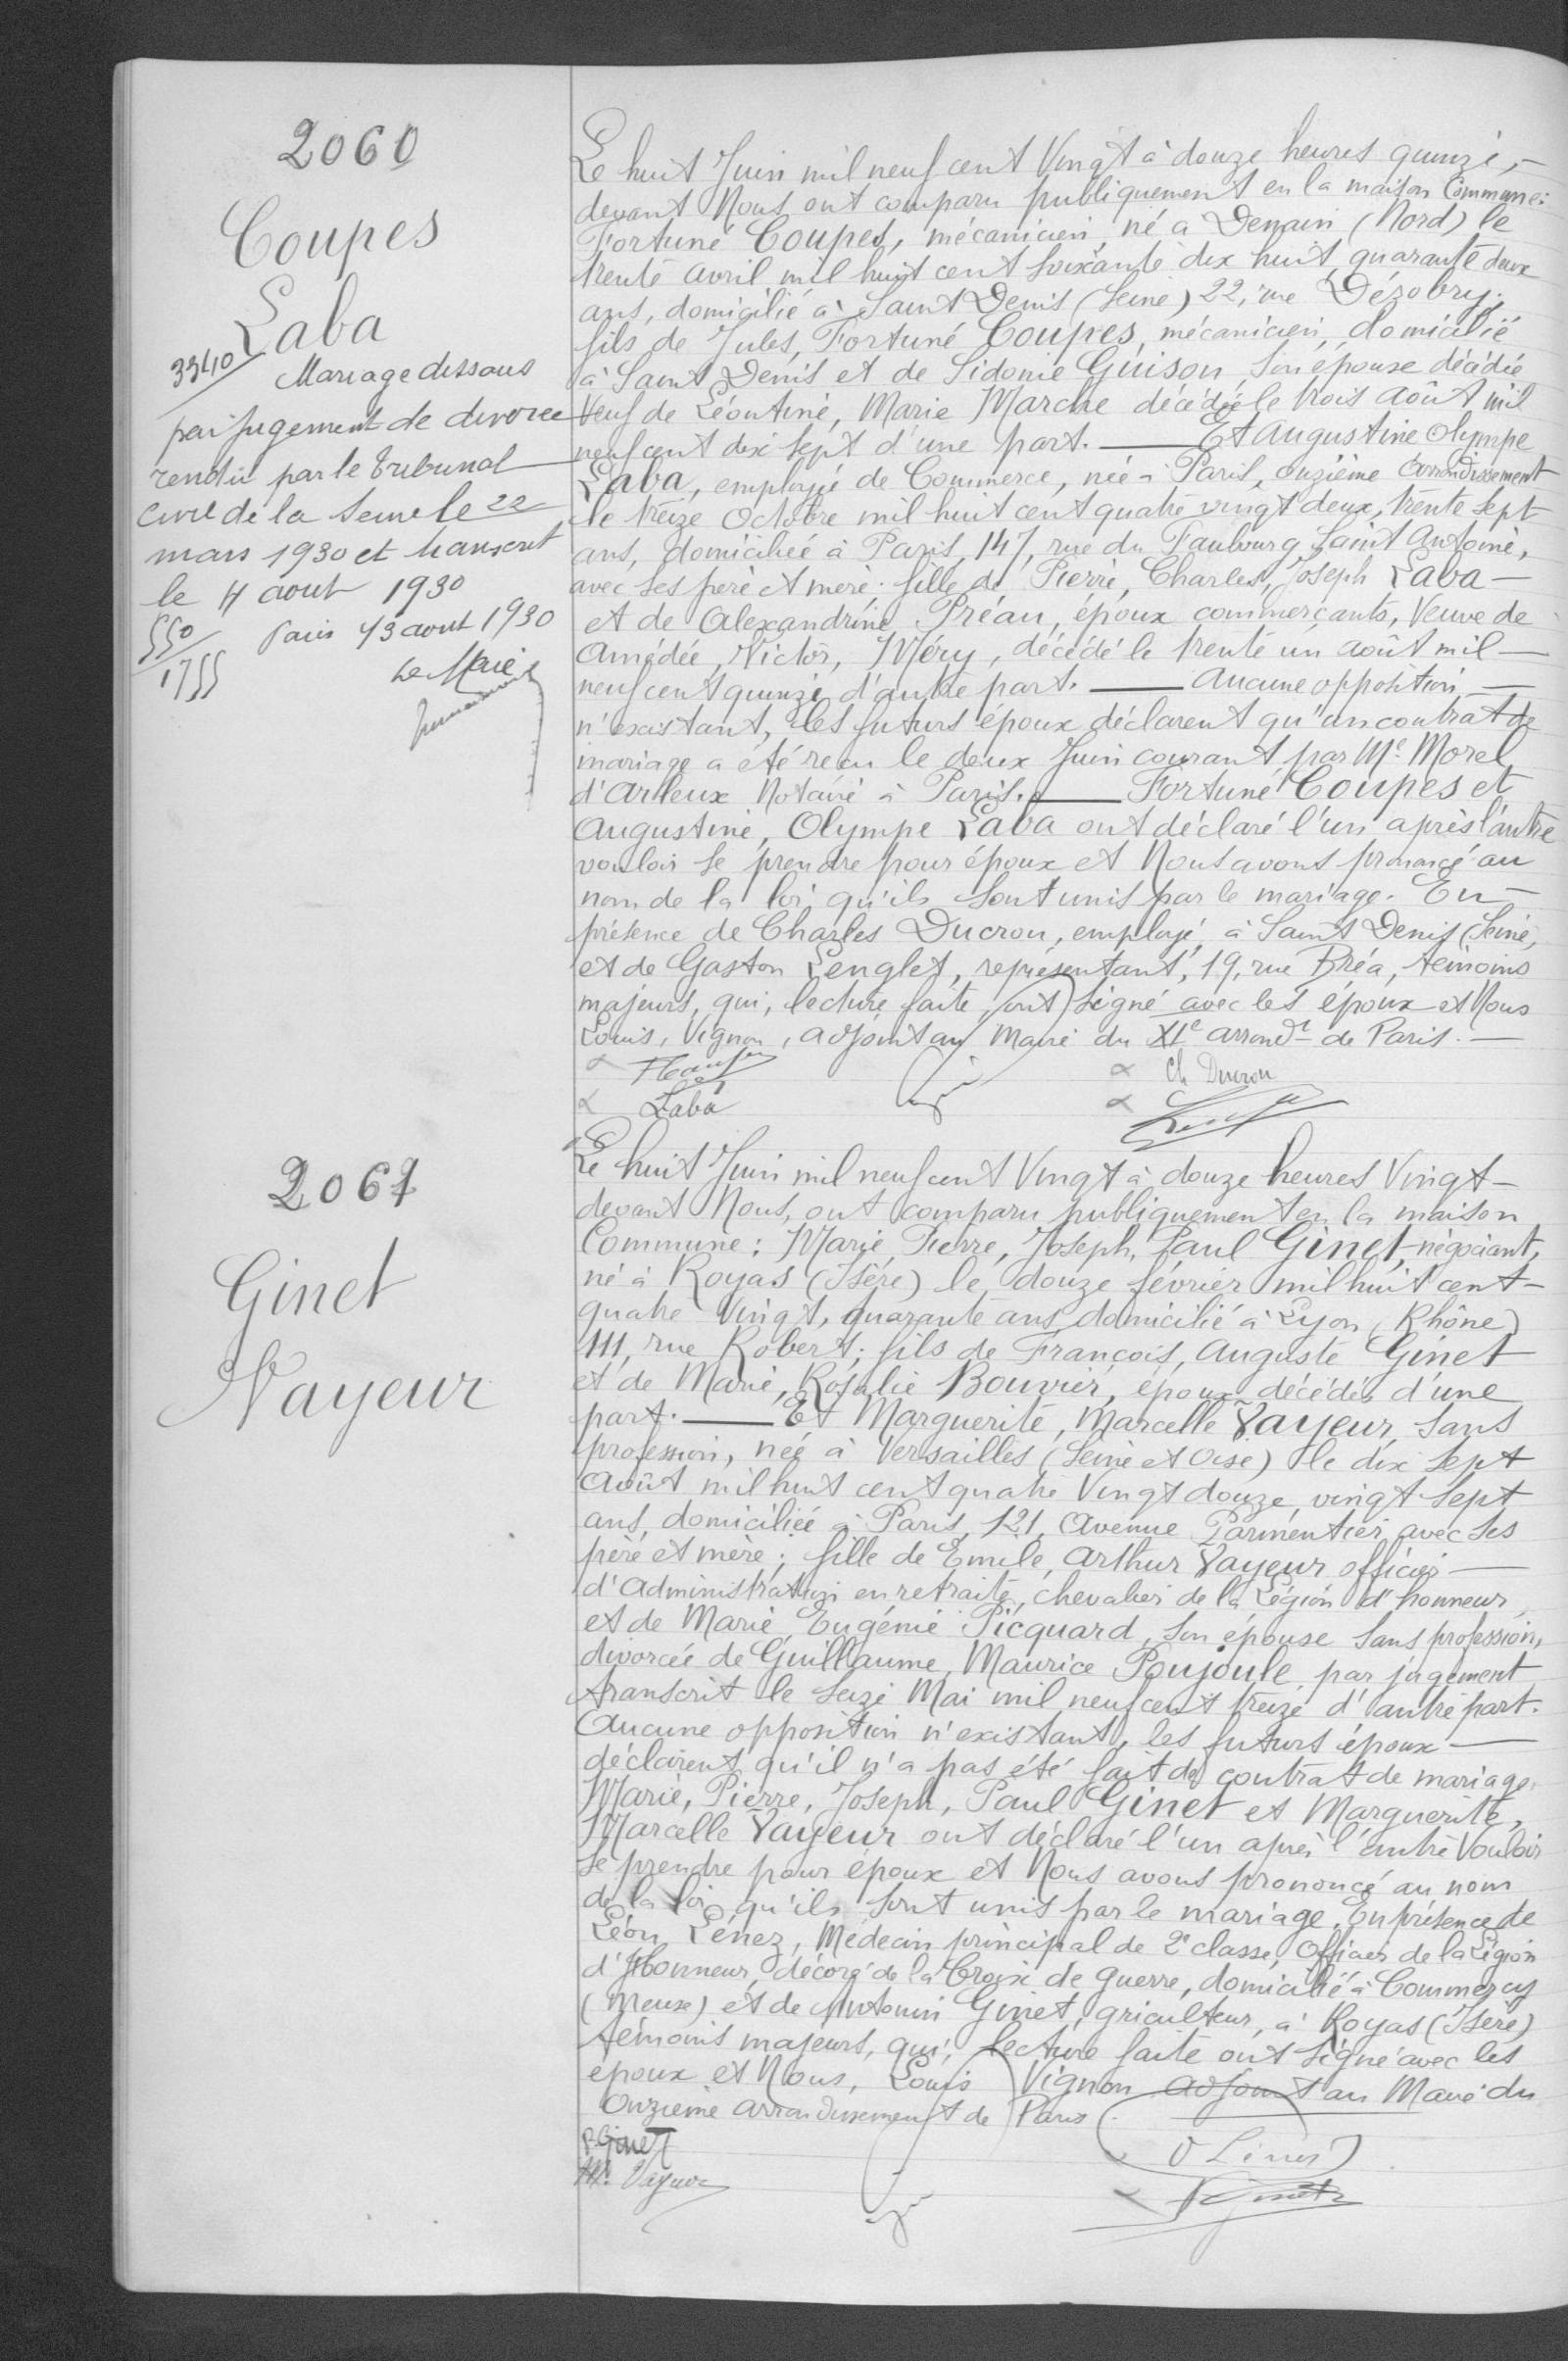2060
Coupes
Laba
Le huit Juin mil neuf cent vingt à douze heures quinze,-
devant Nous ont comparu publiquement en la maison Commune:
Fortuné Coupes, mécanicien, né à Denain (Nord) le
trente avril mil huit cent soixante dix huit, quarante deux
ans, domicilié à Saint Denis (Seine) 22, rue Dézobry;
fils de Jules, Fortuné Coupes, mécanicien, domicilié
à Saint Denis et de Sidonie Guison, son épouse décédée
veuf de Léontine, Marie Marche décédé le trois août mil
neuf cent dix sept d'une part. -Et Augustine Olympe
Laba, employé de Commerce, née à Paris, onzième arrondissement
le treize Octobre mil huit cent quatre vingt deux, trente sept
ans, domicilié à Paris, 147, rue du Faubourg Saint Antoine,
avec ses père et mère; fille de Pierre, Charles, Joseph Laba -
et de Alexandrine Préau, époux commerçants, Veuve de
Amédée Victor, Méry, décédé le trente un août mil -
neuf cent quinze d'autre part. -Aucune opposition -
n'existant, les futurs époux déclarent qu'un contrat de
mariage a été reçu le deux Juin courant par Me Morel
d'Arleux Notaire à Paris. -Fortuné Coupes et
Augustine, Olympe Laba ont déclaré l'un après l'autre
vouloir se prendre pour époux et Nous avons prononcé au
nom de la loi qu'ils sont unis par le mariage. En-
présence de Charles Ducrou, employé à Saint Denis (Seine)
et de Gaston Lengles, représentant, 19, rue Bréa, témoins
majeurs, qui, lecture faite, ont signé avec les époux et Nous
Louis, Vignon, adjoint au Maire du XIe arrond de Paris. -
2067
Ginet
Mayeur
Le huit Juin mil neuf cent Vingt à douze heures Vingt-
devant Nous, ont comparu publiquement en la maison
commune: Marie Pierre, Joeph, Paul Ginet, négociant,
né à Royas (Isère) le douze février mil huit cent-
quatre vingt, quarante ans, domicilié à Lyon (Rhône)
111, rue Robert; fils de François Auguste Ginet
et de Marie, Rosalie Bouvier, époux décédés d'une
part.-Et Marguerite, Marcelle Vayeur, Sans
profession, née à Versailles (Seine et Oise) le dix sept
Août mil huit cent quatre vingt douze, vingt sept
ans, domiciliée à Paris, 121, Avenue Parmentier avec ses
père et mère; fille de Emile, Arthur, Vayeur, officier -
d'Administration en retraite, chevalier de la Légion d'Honneur
et de Marie Eugénie Picquard, son épouse sans profession,
divorcée de Guillaume Maurice Poujoule, par jugement
transcrit le seize Mai mil neuf cent treize d'autre part.
Aucune opposition n'existant, les futurs époux -
déclarent qu'il n'a pas été fait de contrat de mariage
Marie, Pierre, Joseph, Paul Ginet et Marguerite,
Marcelle Vayeur ont déclaré l'un après l'autre vouloir
se prendre pour époux et Nous avons prononcé au nom
de la loi qu'ils sont unis par le mariage. En présence de
Léon Lénez, Médecin principal de 2e classe, Officier de la Légion
d'Honneur, décoré de la Croix de Guerre, domicilié à Commercy
(Meuse) et de Antonin Ginet, agriculteur, à Royas (Isère)
témoins majeurs, qui, lecture faite ont signé avec les
epoux et Nous, Louis Vignon, adjoint au Maire du
Onzième arrondissement de Paris
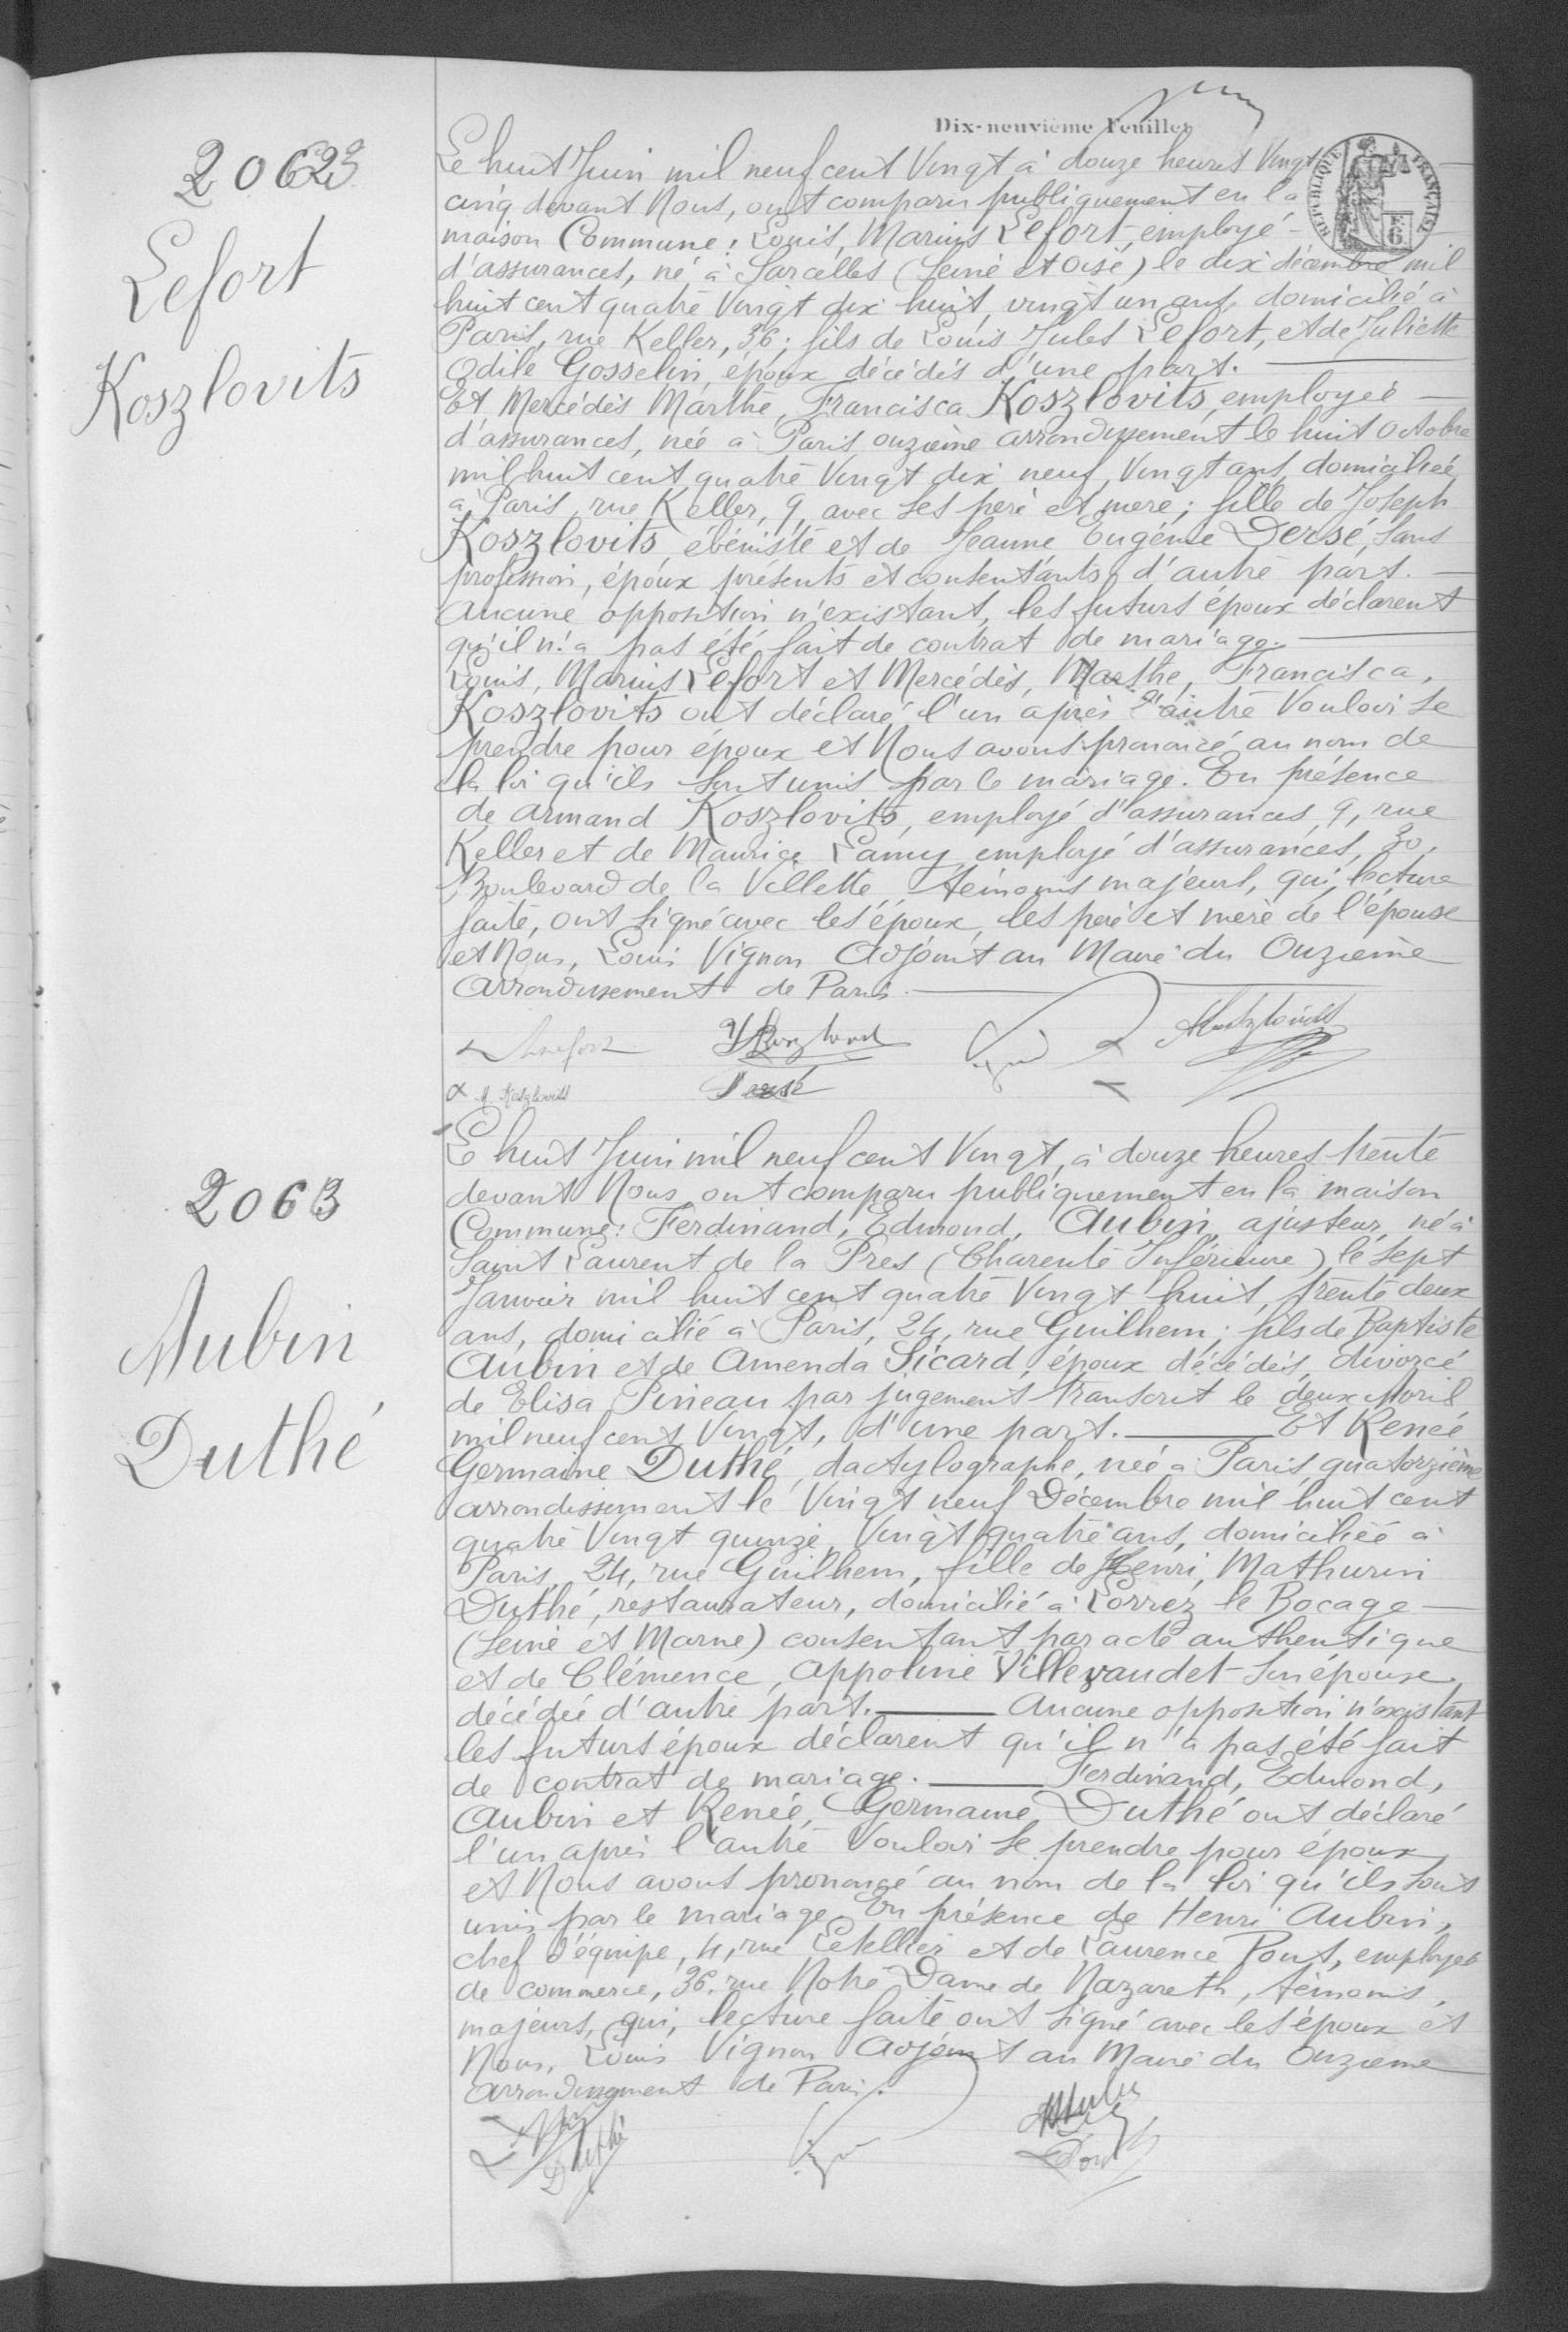2062
Lefort
Koszlovits
Le huit Juin mil neuf cent vingt à douze heures vingt
cinq devant Nous, ont comparu publiquement en la
maison Commune: Louis Marius Lefort, employé
d'assurances, né à Sarcelles (Seine et Oise) le dix décembre mil
huit cent quatre vingt dix huit, vingt un ans, domicilié à
Paris, rue Keller, 36; fils de Louis Jules Lefort, et de Juliette
Odile Gosselin, époux décédés d'une part. -
Et Mercédès Marthe Francisca Koszlovits, employée -
d'assurances, née à Paris onzième arrondissement le huit Octobre
mil huit cent quatre Vingt dix neuf, Vingt ans domiciliée
à Paris rue Keller, 9, avec ses père et mère; fille de Joseph
Koszlovits, ébéniste et de Jeanne Eugénie Dersé, sans
profession, époux présents et consentants d'autre part.-
Aucune opposition n'existant, les futurs époux déclarent
qu'il n'a pas été fait de contrat de mariage. -
Louis, Marius Lefort et Mercédès, Marthe, Francisca,
Koszlovits ont déclaré l'un après l'autre vouloir se
prendre pour époux et Nous avons prononcé au nom de
la loi qu'ils sont unis par le mariage. En présence
de Armand Koszlovitz, employé d'assurances, 9, rue
Keller et de Maurice Lamy, employé d'assurances, 30
Boulevard de la Villette, témoins majeurs, qui, lecture
faite, ont signé avec les époux, les père et mère de l'épouse
et Nous, Louis Vignon Adjoint au Maire du Onzième
Arrondissement de Paris.
2063
Aubin
Duthé
Le huit Juin mil neuf cent Vingt, à douze heures trente
devant Nous ont comparu publiquement en la maison
Commune: Ferdinand, Edmond, Aubin, ajusteur, né à
Saint Laurent de la Pres (Charente Inférieure) le sept
Janvier mil huit cent quatre vingt huit, trente deux
ans, domicilié à Paris, 24, rue Guilhem; fils de Baptiste
Aubin et de Amenda Sicard, époux décédés, divorcé
de Elisa Pineau par jugement transcrit le deux Avril,
mil neuf cent vingt, d'une part.-Et Renée
Germaine Duthé, dactylographe, née à Paris, quatorzième
arrondissement le Vingt neuf Décembre mil huit cent
Quatre Vingt quinze, vingt quatre ans, domiciliée à
Paris, 24, rue Guilhem, fille de Henri, Mathurin
Duthé, restaurateur, domicilié à Lorrez le Bocage
(Seine et Marne) consentant par acte authentique
et de Clémence, Appoline Villevaudet Son épouse,
décédée d'autre part. -Aucune opposition n'existant
les futurs époux déclarent qu'il n'a pas été fait
de contrat de mariage.-Ferdinand, Edmond,
Aubin et Renée, Germaine, Duthé ont déclaré
l'un après l'autre vouloir se prendre pour époux,
et Nous avons prononcé au nom de la loi qu'ils sont
unis par le mariage. En présence de Henri Aubin,
chef d'équipe, 4, rue Letellier et de Laurence Pont, employée
de commerce, 36 rue Notre Dame de Nazaret, témoins
majeurs, qui, lecture faite ont signé avec les époux et
Nous, Louis Vignon Adjoint au Maire du Onzième
Arrondissement de Paris.